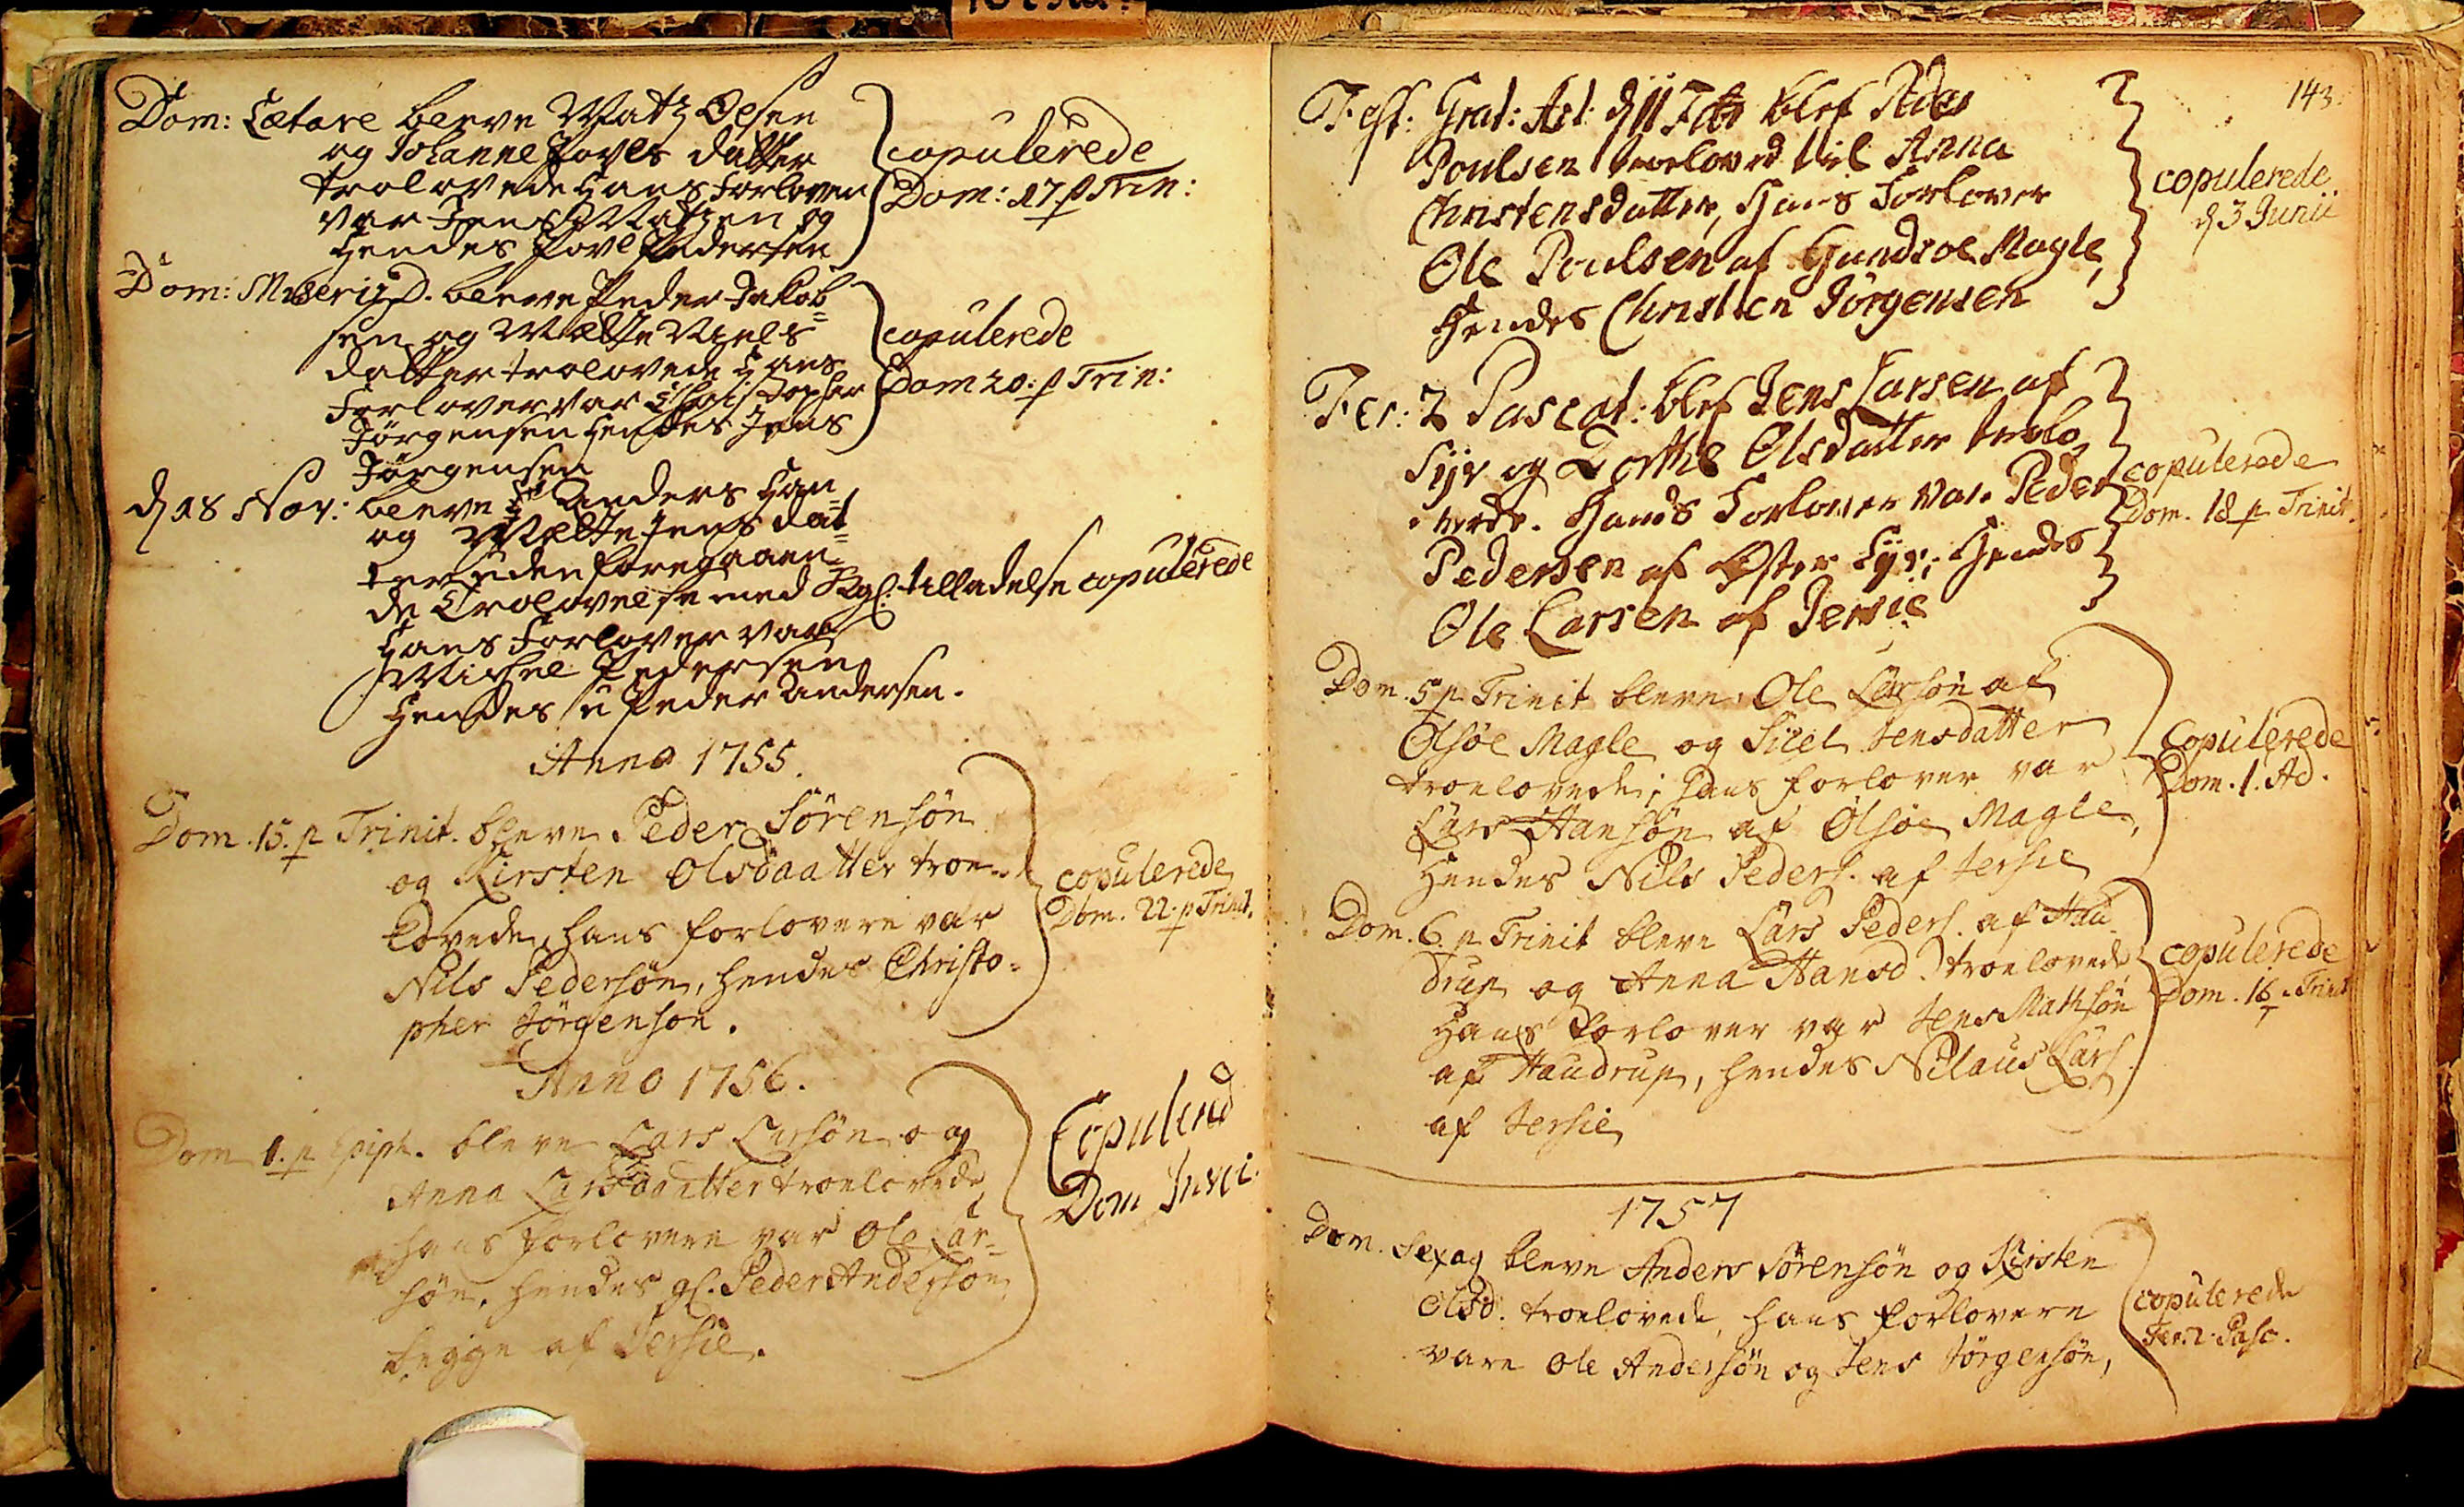Dom: Lætare blev Matz Olsen
og Johanne Povls datter
trolovede Hans Forlover
var Jens Matzen og
Hendes Povl Pedersen
Copulerede
Dom: 17. p Trin:
Dom: Miseric D. blev Peder Jacob¬
sen og Mætte Niels
datter trolovede Hans
Forlover var Christopher
Jørgensen Hendes Jens
Jørgensen
Copulerede
Dom 20: p Trin:
d 18 Nov: bleve ## Anders Han¬
og Mætte Jens dat¬
ter uden foregaaen¬
de Trolovelse med Kgl: tilladelse copulerede
Hans Forlover vare
Michel Pedersen
Hendes u Peder Andersen.
Anno 1755.
Dom. 15. p Trinit bleve Peder Sørensøn
og Kirsten Olsdaatter troe¬
lovede, hans forlovere var
Nils Pedersøn, hendes Christo¬
pher Jørgensøn.
Copulerede
Dom. 22. p Trinit.
Anno 1756.
Dom 1. p Epiph. bleve Lars Larsøn og
Anna Larsdaatter troelovede
hans forlovere var Ole Lar¬
søn, hendes gl. Peder Andersøn
begge af Jersie.
Copulered
Dom Invoc.
143.
Fest: Grat: Act: d 11 Febr blef Peder
Poulsen troloved til Anna
Christensdatter, Hans forlover
Ole Poulsen af Gundsøe Magle,
Hendes Christen Jørgensen
Copulerede
d 3 Junii
Fer: 2 Pascat: blef Jens Larsen af
Syv og Dorthe Olsdatter trolo¬
-vede. Hands Forlover var. Peder
Pedersen af Øster Syv, Hendes
Ole Larsen af Jersie
Copulerede
Dom. 18 p Trinit.
Dom. 5 p Trinit bleve Ole Larsøn af
Ølsøe Magle og Sicel Jensdatter
trolovede; hans forlover var
Lars Hansøn af Ølsøe Magle,
Hendes Nils Peders. af Jersie
Copulerede
Dom. 1. Ad.
Dom. 6. p Trinit bleve Lars Peders. af Hau¬
drup og Anna Hansd. troelovede
Hans forlover var Jens Mathsøn
af Haudrup, hendes Nilaus Lars.
af Jersie
Copulerede
Dom. 16 p Trinit
1757
Dom. Sexag bleve Anders Sørensøn og Kirsten
Olsd. troelovede, hans forlovere
vare Ole Andersøn og Jens Jørgensøn,
Copulerede
Fer. 2. Pasc.
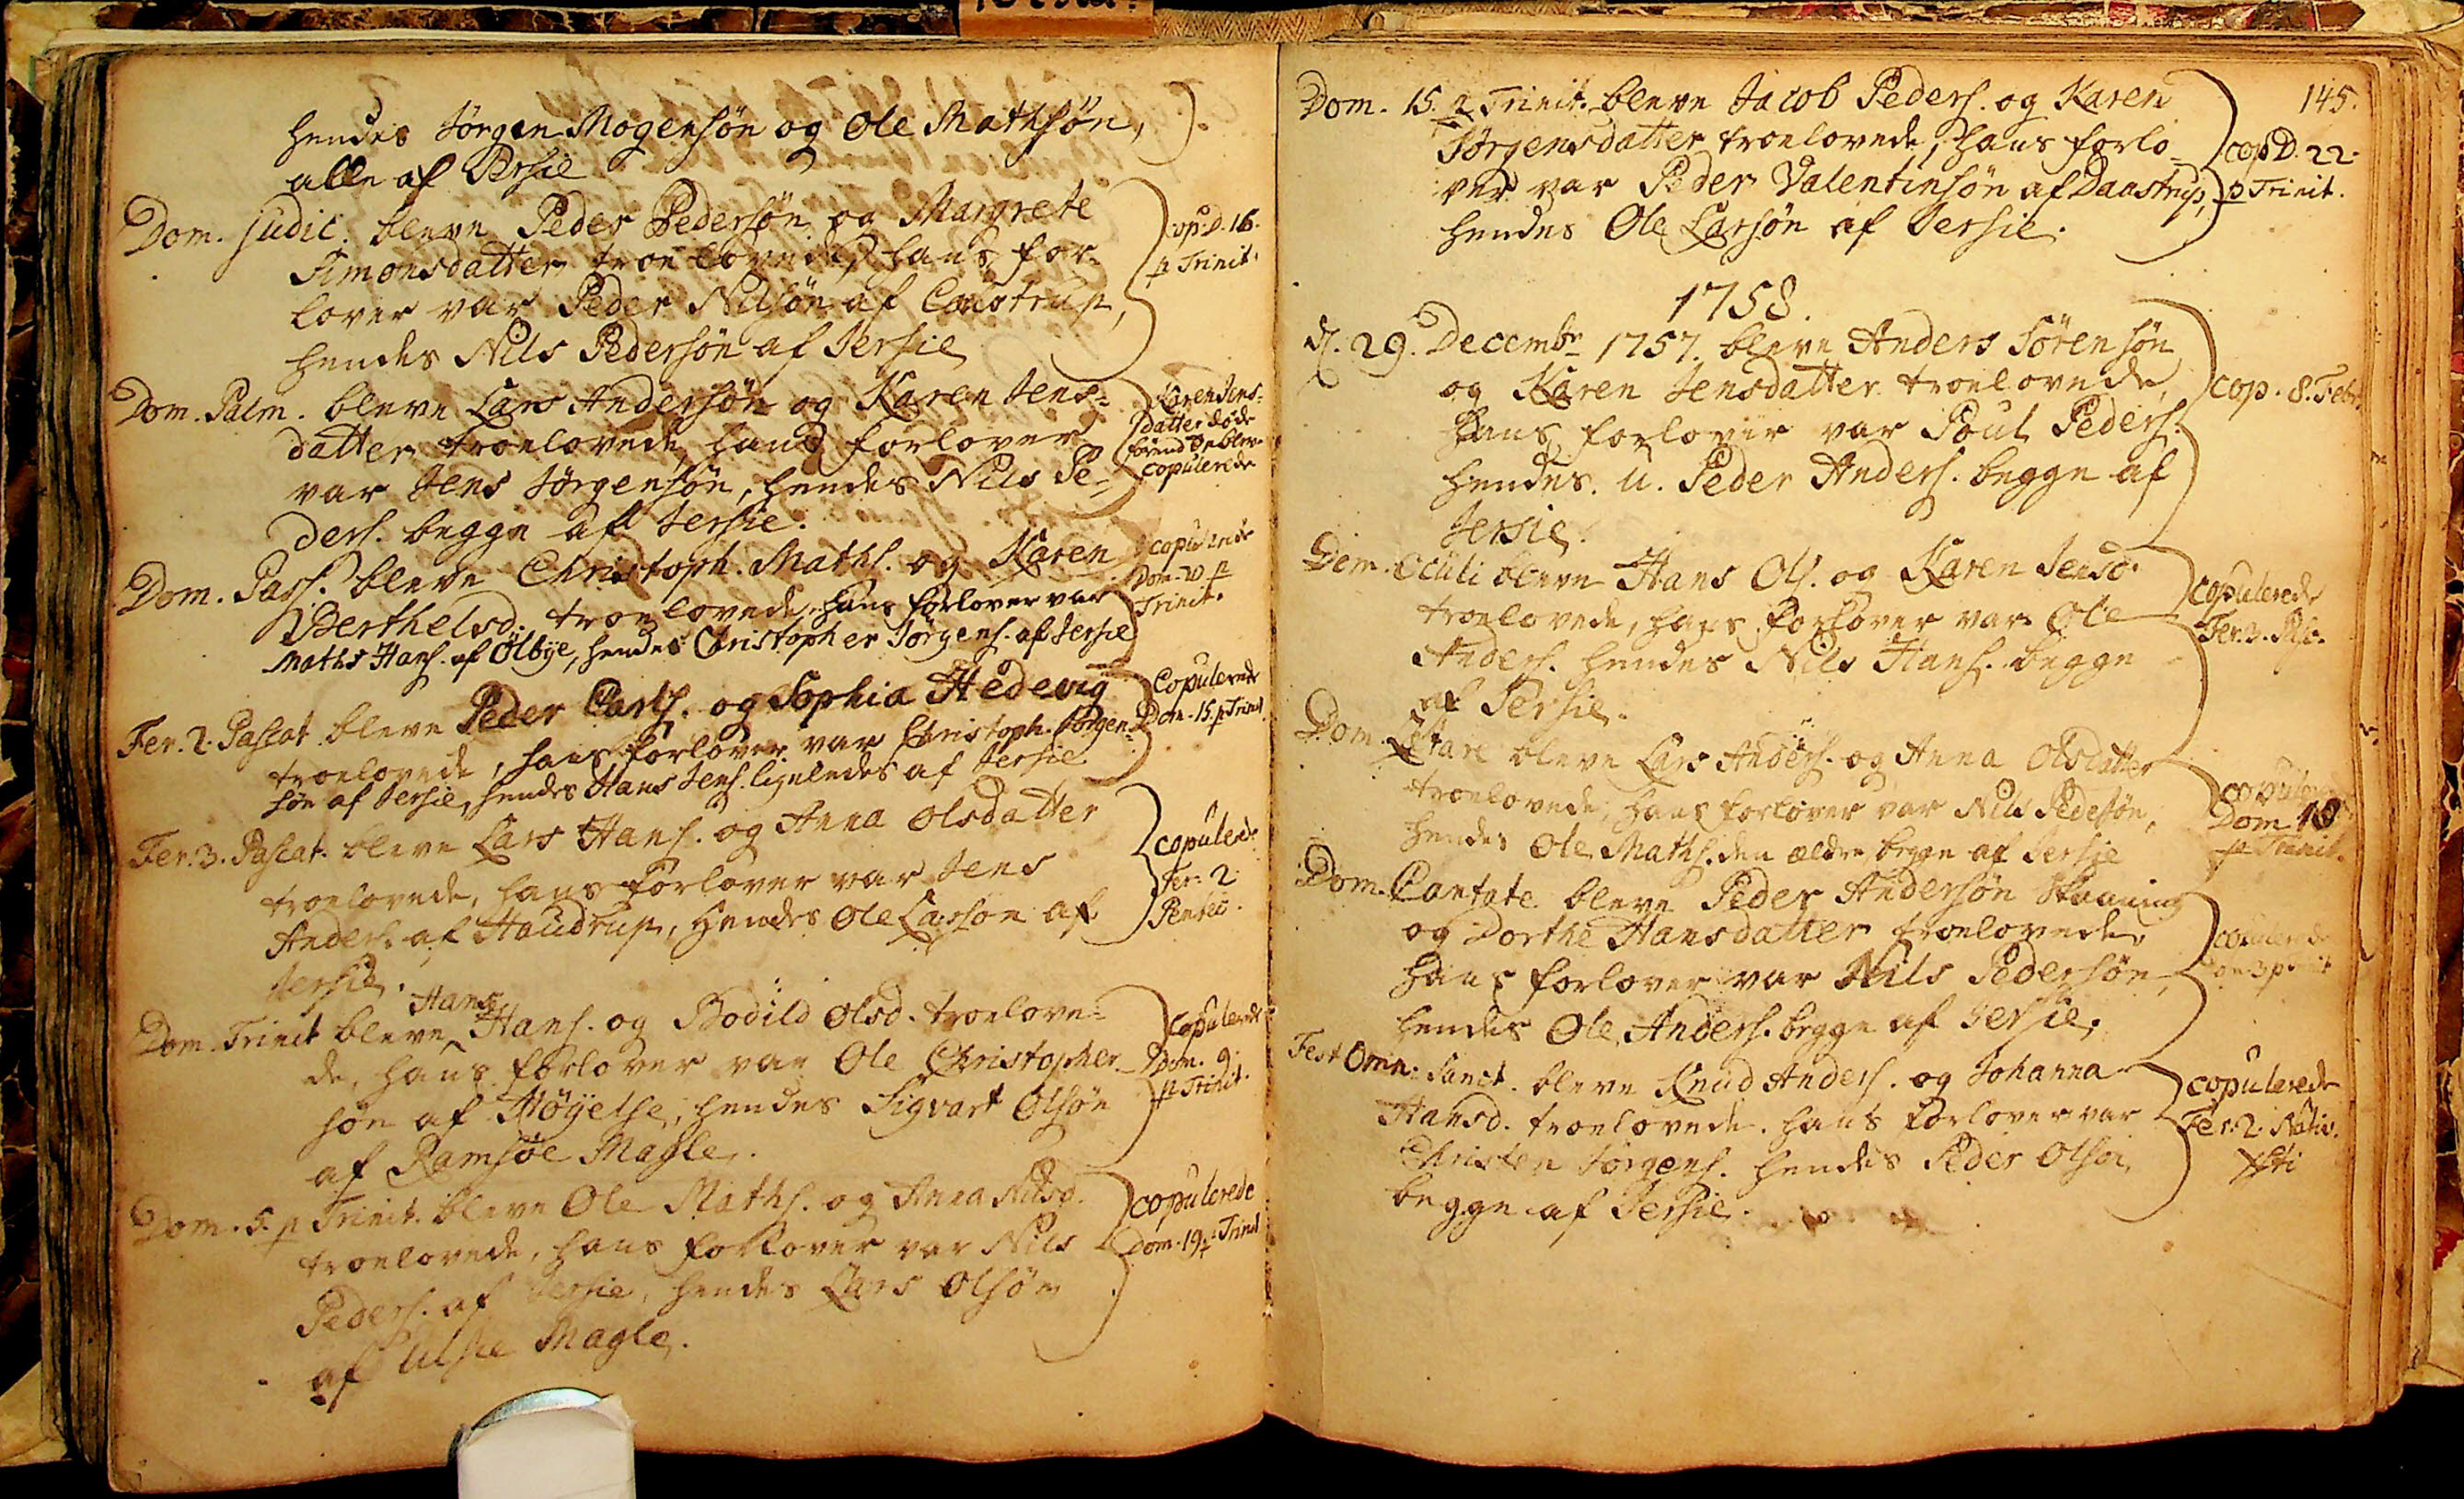hendes Jørgen Mogensøn og Ole Mathsøn,
alle af Jersie
Dom. Judic. bleve Peder Pedersøn og Margrete
Simonsdatter troelovede, hans for¬
lover var Peder Nielsøn af Carlstrup,
hendes Nils Pedersøn af Jersie
Cop: D. 16.
p Trinit:
Dom. Palm. bleve Lars Andersøn og Karen Jens¬
datter troelovede, hans forlover
var Jens Jørgensøn, hendes Nils Pe¬
ders. begge af Jersie.
Karen Jens¬
datter døde
førend de blev
Copulerede
Dom. Pass: bleve Christoph. Maths. og Karen
Berthelsd. troelovede, hans forlover var
Maths Hans. af Ølbye, hendes Christopher Jørgens. af Jersie
Copulerede
Dom. 20 p
Trinit.
Fer. 2. Pascat bleve Peder Carls. og Sophia Hedevig
troelovede, hans forlover var Christopher Jørgen¬
søn af Jersie, hendes Hans Jens. ligeledes af Jersie
Copulerede
Dom.15.p Trinit
Fer. 3. Pascat. bleve Lars Hans. og Anna Olsdatter
troelovede, hans forlover var Jens
Anders. af Haudrup, Hendes Ole Larsøn af
Jersie.
Copulerede
Fer: 2.
Pentec.
Hans
Dom. Trinit bleve Hans. og Bodild Olsd. troelove¬
de, hans forlover var Ole Christopher
søn af Høyelse, hendes Sigvart Olsøn
af Ramsøe Magle.
Copulerede
Dom. 9.
p Trinit.
Dom. 5. p Trinit. bleve Ole Maths. og Anna Nilsd.
troelovede, hans forlover var Nils
Peders. af Jersie, hendes Lars Olsøn
af Ulsie Magle.
Copulerede
Dom.19.p Trinit
145.
Dom. 15. p Trinit. bleve Jacob Peders. og Karen
Jørgensdatter troelovede, hans forlo¬
ver var Peder Valentinsøn af Daastrup,
hendes Ole Larsøn af Jersie.
Cop D. 22.
p Trinit.
1758.
d. 29. Decembr 1757. bleve Anders Sørensøn
og Karen Jensdatter troelovede,
hans forlover var Poul Peders.
hendes. u. Peder Anders. begge af
Jersie.
Cop. 8. Febr.
Dom. Oculi bleve Hans Ols. og Karen Jensd.
troelovede, hans forlover var Ole
Anders. hendes Nils Hans. begge
af Jersie.
Copulerede
Fer. 3. Pasc.
Dom. Lætare bleve Lars Anders. og Anna Olsdatter
troelovede, hans forlover var Nils Pedersøn,
hendes Ole Maths. den ældre, begge af Jersie
Copulerede
Dom 18##
p Trinit.
Dom. Cantate bleve Peder Andersøn Skaaning
og Dorthe Hansdatter troelovede
hans forlover var Nils Pedersøn,
hendes Ole Anders. begge af Jersie.
Copulerede
Dom. 3 p Trinit
Fest Omn. Sanct. bleve Knud Anders. og Johanna
Hansd. troelovede. hans forlover var
Christen Jørgens. hendes Peder Olsøn.
begge af Jersie.
Copulerede
Fer: 2. Nativ.
Xsti